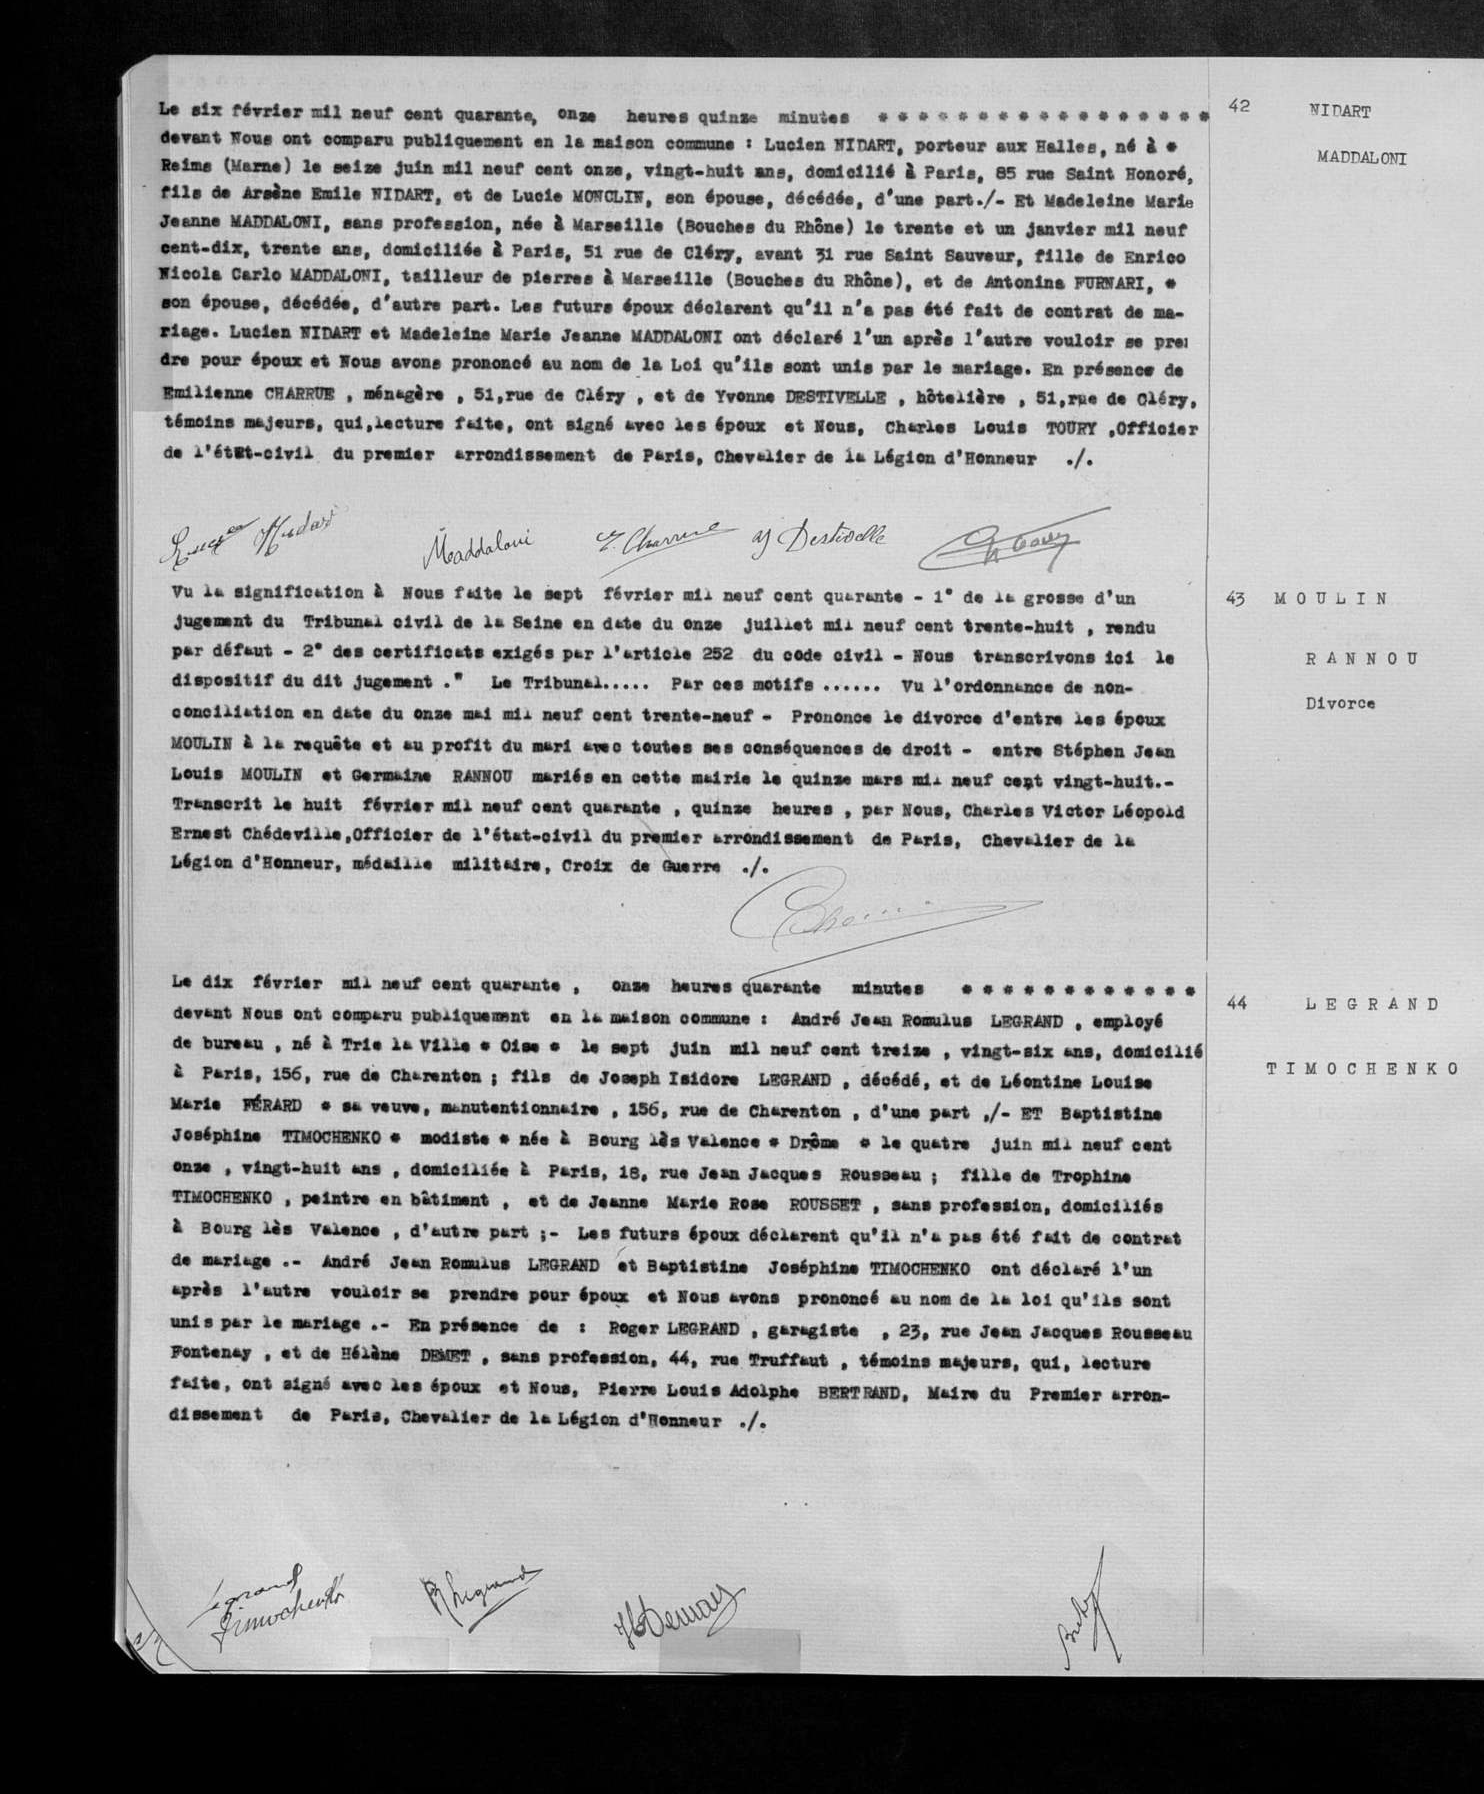Le six février mil neuf cent quarante, onze heures quinze minutes ****
devant Nous ont comparu publiquement en la maison commune: Lucien NIDART, porteur aux Halles, né à *
Reims (Marne) le seize juin mil neuf cent onze, vingt-huit ans, domicilié çà Paris, 85 rue Saint Honoré,
fils de Arsène Emile NIDART, et de Lucie MONCLIN, son épouse, décédée, d'une part ./-Et Madeleine Marie
Jeanne MADDALONI, sans profession, née à Marseille (Bouches du Rhône le trente et un janvier mil neuf
cent-dix, trente ans, domiciliée à Paris, 51 rue de Cléry, avant 31 rue Saint Sauveur, fille de Enrico
Nivola Carlo MADDALONI, tailleur de pierres à Marseille (Bouches du Rhône), et de Antonina FURNARI, *
son épouse, décédée, d'autre part. Les futurs époux déclarent qu'il n'a pas été fait de contrat de ma-
riage. Lucien NIDART et Madeleine Maria Jeanne MADDALONI ont déclaré l'un après l'autre vouloir se pren
dre pour époux et Nous avons prononcé au nom de la lOI qu'ils sont unis par le mariage. En présence de
Emilienne CHARRUE, ménage, 51, rue de Cléry, et de Yvonne DESTIVELLE, hôtelière, 51, rue de Cléry,
témoins majeurs, qui, lecture faite, ont signé avec les époux et Nous, Charles Louis TOURY, Officier
de l'état-civil du premier arrondissement de Paris, Chevalier de la Légion d'Honneur ./.
Vu la signification à nous faite le sept février mil neuf cent quarante-1° de la grosse d'un
jugement du Tribunal civil de la Seine en date du onze juillet mil neuf cent trente-huit, rendu
par défaut-2° des certificats exigés par l'article 252 du code civil-Nous transcrivons ici le
dispositif du dit jugement:" le Tribunal..... Par ces motifs..... Vu l'ordonnance de non-
conciliation en date du onze mai mil neuf cent trente-neuf-Prononce le divorce d'entre les époux
MOULIN à la requête et au profit du mari avec toutes ses conséquences de droit-entre Stéphen Jean
Louis MOULIN et Germaine RANNOU mariés en cette maire le quinze mars mil neuff cent vingt-huit .-
Transcrit le huit février mil neuf cent quarante, quinze heures, par Nous, Charles victor Léopold
Ernest Chédeville Officier de l'état-civil du premier arrondissement de Paris, Chevalier de la
Légion d'Honneur, médaille militaire, Croix de Guerre ./.
Le dix février mil neuf cent quarante, onze heures quarante minutes ****
devant Nous ont comparu publiquement en la maison commune: André Jean Romulus LEGRAND, employé
de bureau, né à Trie la Ville * Oise * le sept juin mil neuf cent treize, vingt-six ans, domicilié
à Paris, 156, rue de Charenton; fils de Joseph Isidore LEGRAND, décédé et de Léontine Louise
Marie FERARD * sa veuve, manutentionnaire, 156, rue de Charenton, d'une part ,/-ET Baptistine
Joséphine TIMOCHENKO * modiste * née à Bourg lès Valence * Drôme * le quatre juin mil neuf cent
onze, vingt-huit ans, domiciliée à Paris, 18, rue jean Jacques Rousseau; fille de Trophine
TIMOCHENKO, peintre en bâtiment, et de Jeanne Marie Rose ROUSSET, sans profession, domiciliés
à Bourg lès Valences, d'autre part;-Les futurs époux déclarent qu'il n'a pas été fait de contrat
de mariage .-André Jean Romulus LEGRAND et Baptistine Joséphine TIMOCHENKO ont déclaré l'un
après l'autre vouloir se prendre pou époux et Nous avons prononcé au nom de la loi qu'ils sont
unis par le mariage.-En présence de: Roger LEGRAND ,, garagiste, 23, rue Jean Jacques Rousseau
Fontenay, et de Hélène DEMET, sans profession, 44, rue Truffaut, témoins majeurs, qui, lecture
faite, ont signé avec les époux et Nous, Pierre Louis Adolphe BERTRAND, Maire du Premier arron-
dissident de Paris, Chevalier de la Légion d'Honneur ./.
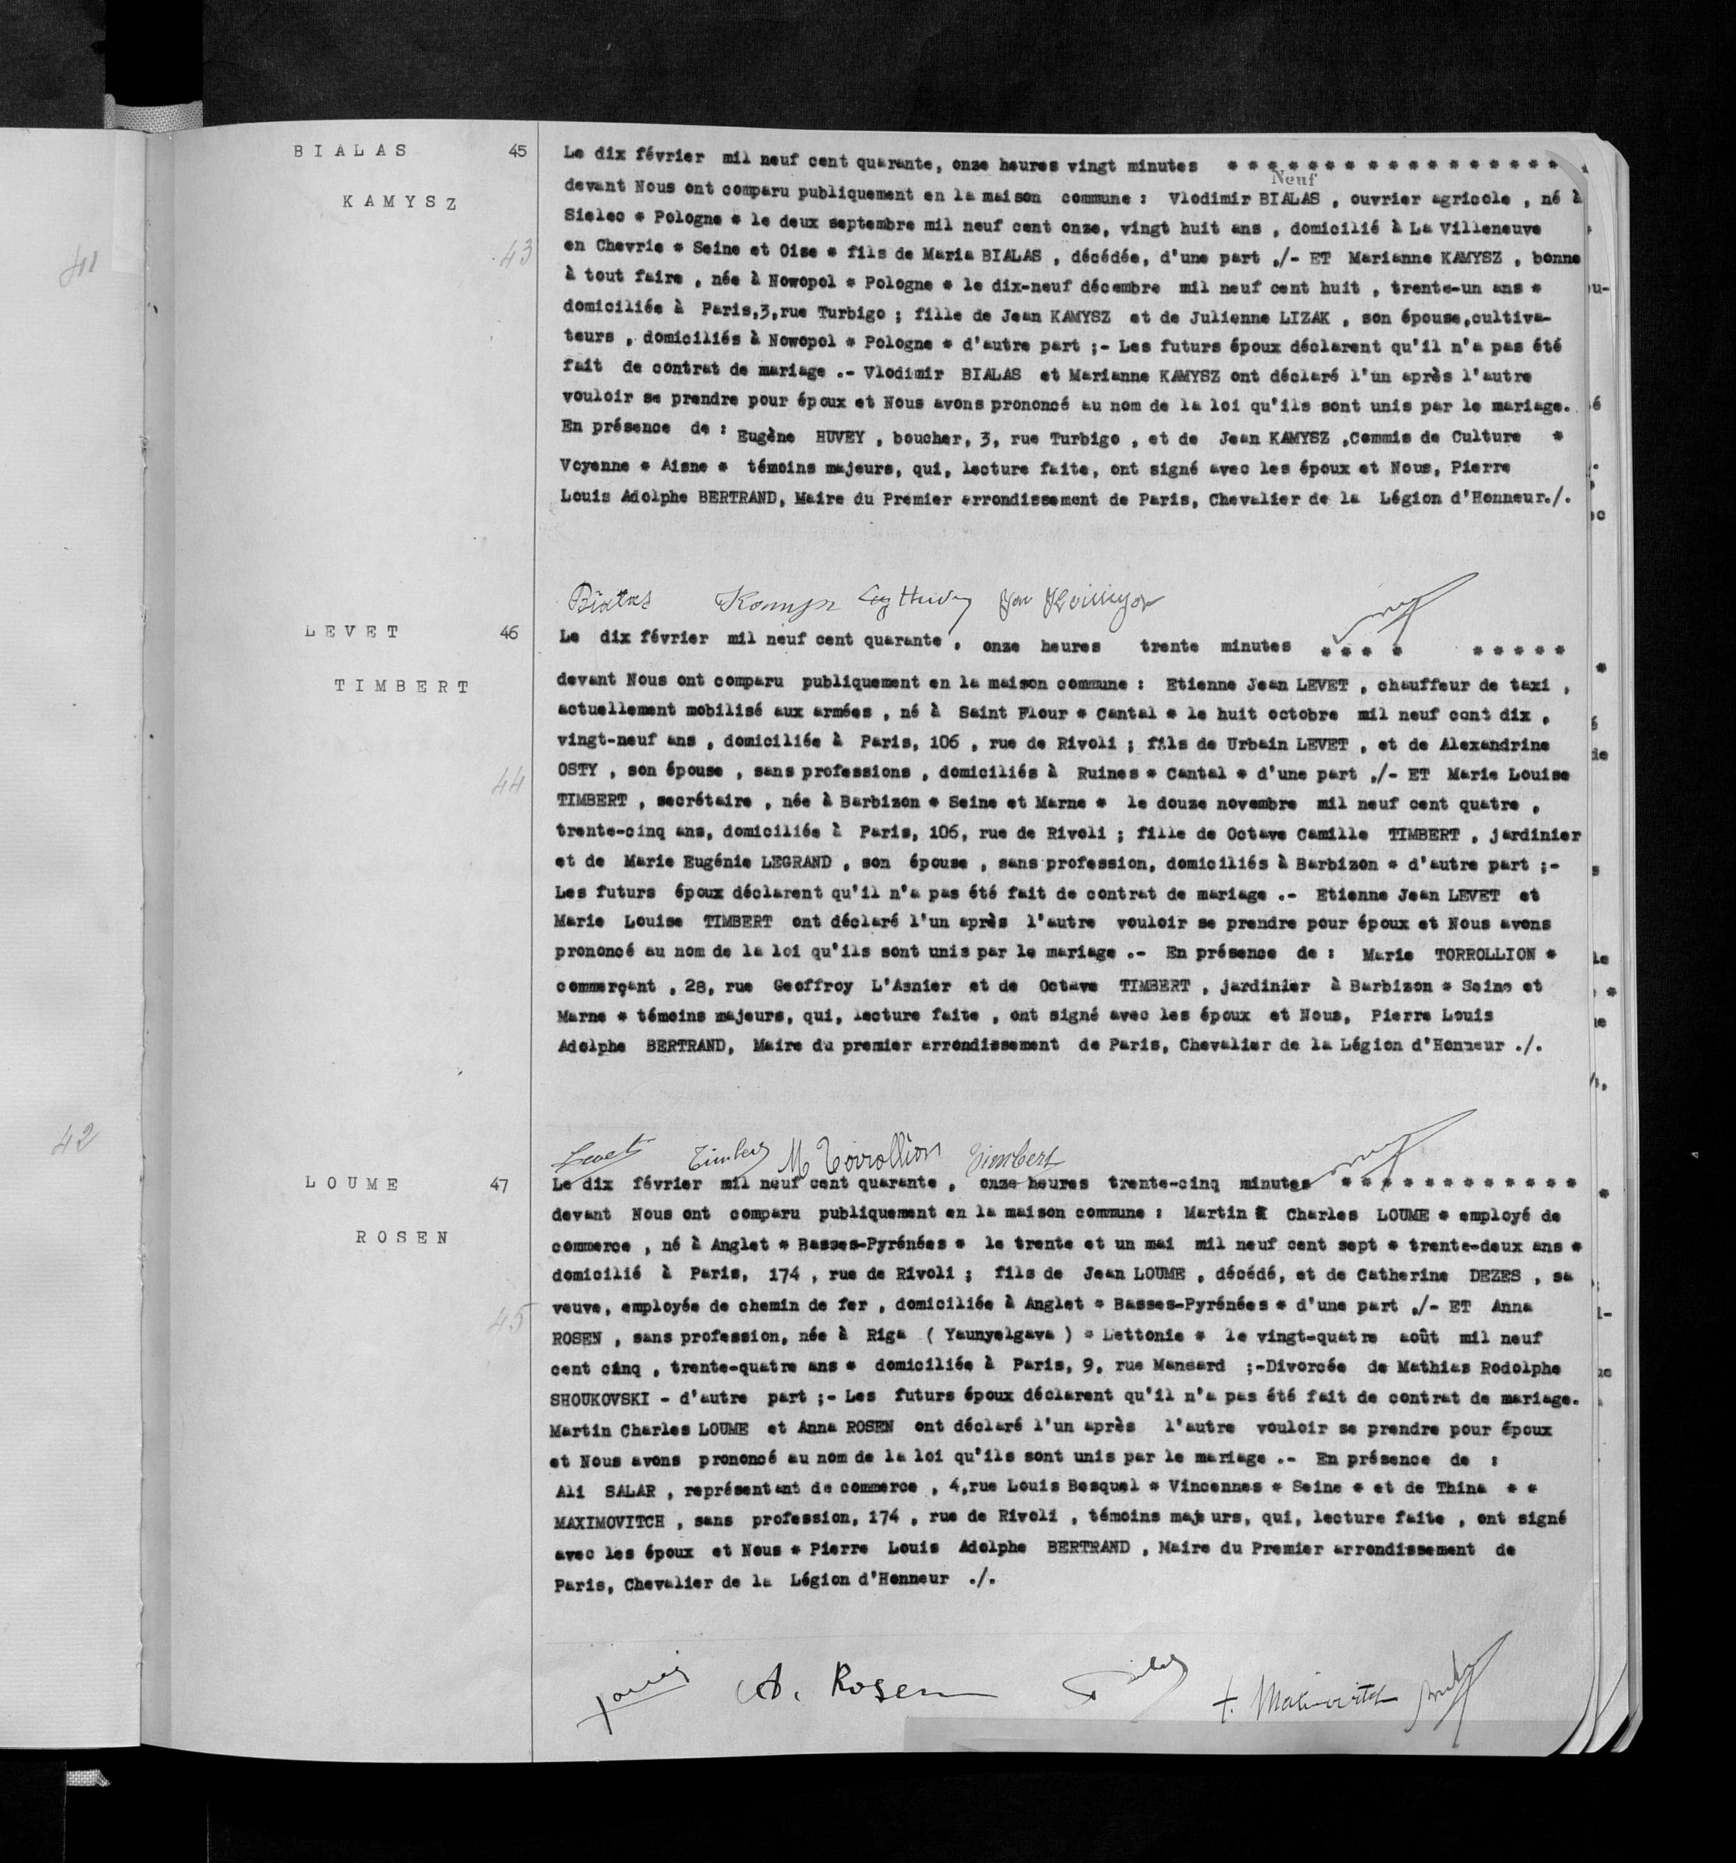Le dix février mil neuf cent quarante, onze heures vingt minutes ****
devant Nous ont comparu publiquement en la maison commune: Vladimir BIALAS, ouvrier agricole, né à
Sieleo * Pologne * le deux septembre mil neuf cent onze, vingt huit ans, domicilié à La Villeneuve
en Chevrie * Seine et Oise * fils de Maria BIALAS, décédée, d'une part ,/-ET Marianne KAMYSZ, bonne
à tout faire, née à Nowopol * Pologne * le dix-neuf décembre mil neuf cent huit, trente-un ans *
domiciliée à Paris, 3, rue Turbigo; fille de Jean KAMISZ et de Julienne LIZAK, son épouse, cultiva-
teurs, domiciliés à Nowopol * Pologne * d'autre part ;-Les futurs époux déclarent qu'il n'a pas été
fait de contrat de mariage .-Vlodimir BIALAS et Marianne KAMYSZ ont déclaré l'un après l'autre
vouloir se prendre pour époux et Nous avons prononcé au nom de la loi qu'ils sont unis par le mariage.
En présence de: Eugène HUVEY, boucher, 3, rue Turbigo, et de Jean KAMYSZ, Commis de Culture *
Voyenne * Aisne * témoins majeurs, qui, lecture faite, ont signé avec les époux et Nous, Pierre
Louis Adolphe BERTRAND, Maire du Premier arrondissement de Paris, Chevalier de la Légion d'Honneur ./.
Le dix février mil neuf cent quarante, onze heures trente minutes ****
devant Nous ont comparu publiquement en la maison commune: Etienne Jean LEVET, chauffeur de taxi,
actuellement mobilisé aux armées, né à Saint Fleur * Cantal * le huit octobre mil neuf cent dix,
vingt-neuf ans, domiciliée à Paris, 106, rue de Rivoli; fils de Urbain LEVET, et de Alexandrine
OSTY, son épouse, sans professions, domiciliés à Ruines * Cantal * d'une part ,/-ET Marie Louise
TIMBERT, secrétaire, née à Barbison * Seine et Marne * le douze novembre mil neuf cent quatre,
trente-cinq ans, domiciliés à Paris, 106, rue de Rivoli; fille de Octave Camille TIMBERT, jardinier
et Marie Eugène LEGRAND, son épouse, sans profession, domiciliés à Barbizon * d'autre part;
Les futurs époux déclarent qu'ils n'a pas été fait de contrat de mariage .-Etienne Jean LEVET et
Marie Louise TIMBERT ont déclaré l'un après l'autre vouloir se prendre pou époux et Nous avons
prononcé au nom de la loi qu'ils sont unis par le mariage .-En présence de: Marie TORROLION *
commerçant, 28, rue Geoffroy L'Asnier et de Octave TIMBERT, jardinier à Barbizon * Seine et
Marne * témoins majeurs, qui, lecture faite, ont signé avec les époux et Nous, Pierre Louis
Adolphe BERTRAND, Maire du premier arrondissement de Paris, Chevalier de la Légion d'Honneur ./.
Le dix février mil neuf cent quarante, onze heures trente-cinq minutes ****
devant Nous ont comparu publiquement en la maison commune: Martin Charles LOUME * employé de
commerce, né à Anglet * Basses-Pyrénées * le trente et un mai mil neuf cent sept * trente-deux ans *
domicilié à Paris, 174, rue de Rivoli; fils de Jean LOUME, décédé, et de Catherine DEZES, sa
veuve, employée de chemin de fer, domicilié à Anglet * Basses-Pyrénées * d'une part ,/-ET Anna
ROSEN, sans profession, née à Riga (Yaunyolgava) * Lettonie * le vingt-quatre août mil neuf
cent cinq, trente-quatre ans * domiciliée à Paris, 9, rue Mansard ;-Divorcée de Mathias Rodolphe
SHOUKOVSKI-d'autre part ;-Les futurs époux déclarent qu'il n'a pas été fait de contrat de mariage.
Martin Charles LOUME et Anna ROSEN ont déclaré l'un après l'autre vouloir se prendre pour époux
et Nous avons prononcé au nom de la loi qu'ils sont unis par le mariage .-En présence de:
Ali SALAR, représentant de commerce, 4, rue louis Besquel * Vincennes * Seine * et de Thina **
MAXIMOVITCH, sans profession, 174, rue de Rivoli, témoins majeurs, qui, lecture faite, ont signé
avec les époux et Nous * Pierre Louis Adolphe BERTRAND, Maire du Premier arrondissement de
Paris, Chevalier de la Légion d'Honneur ./.